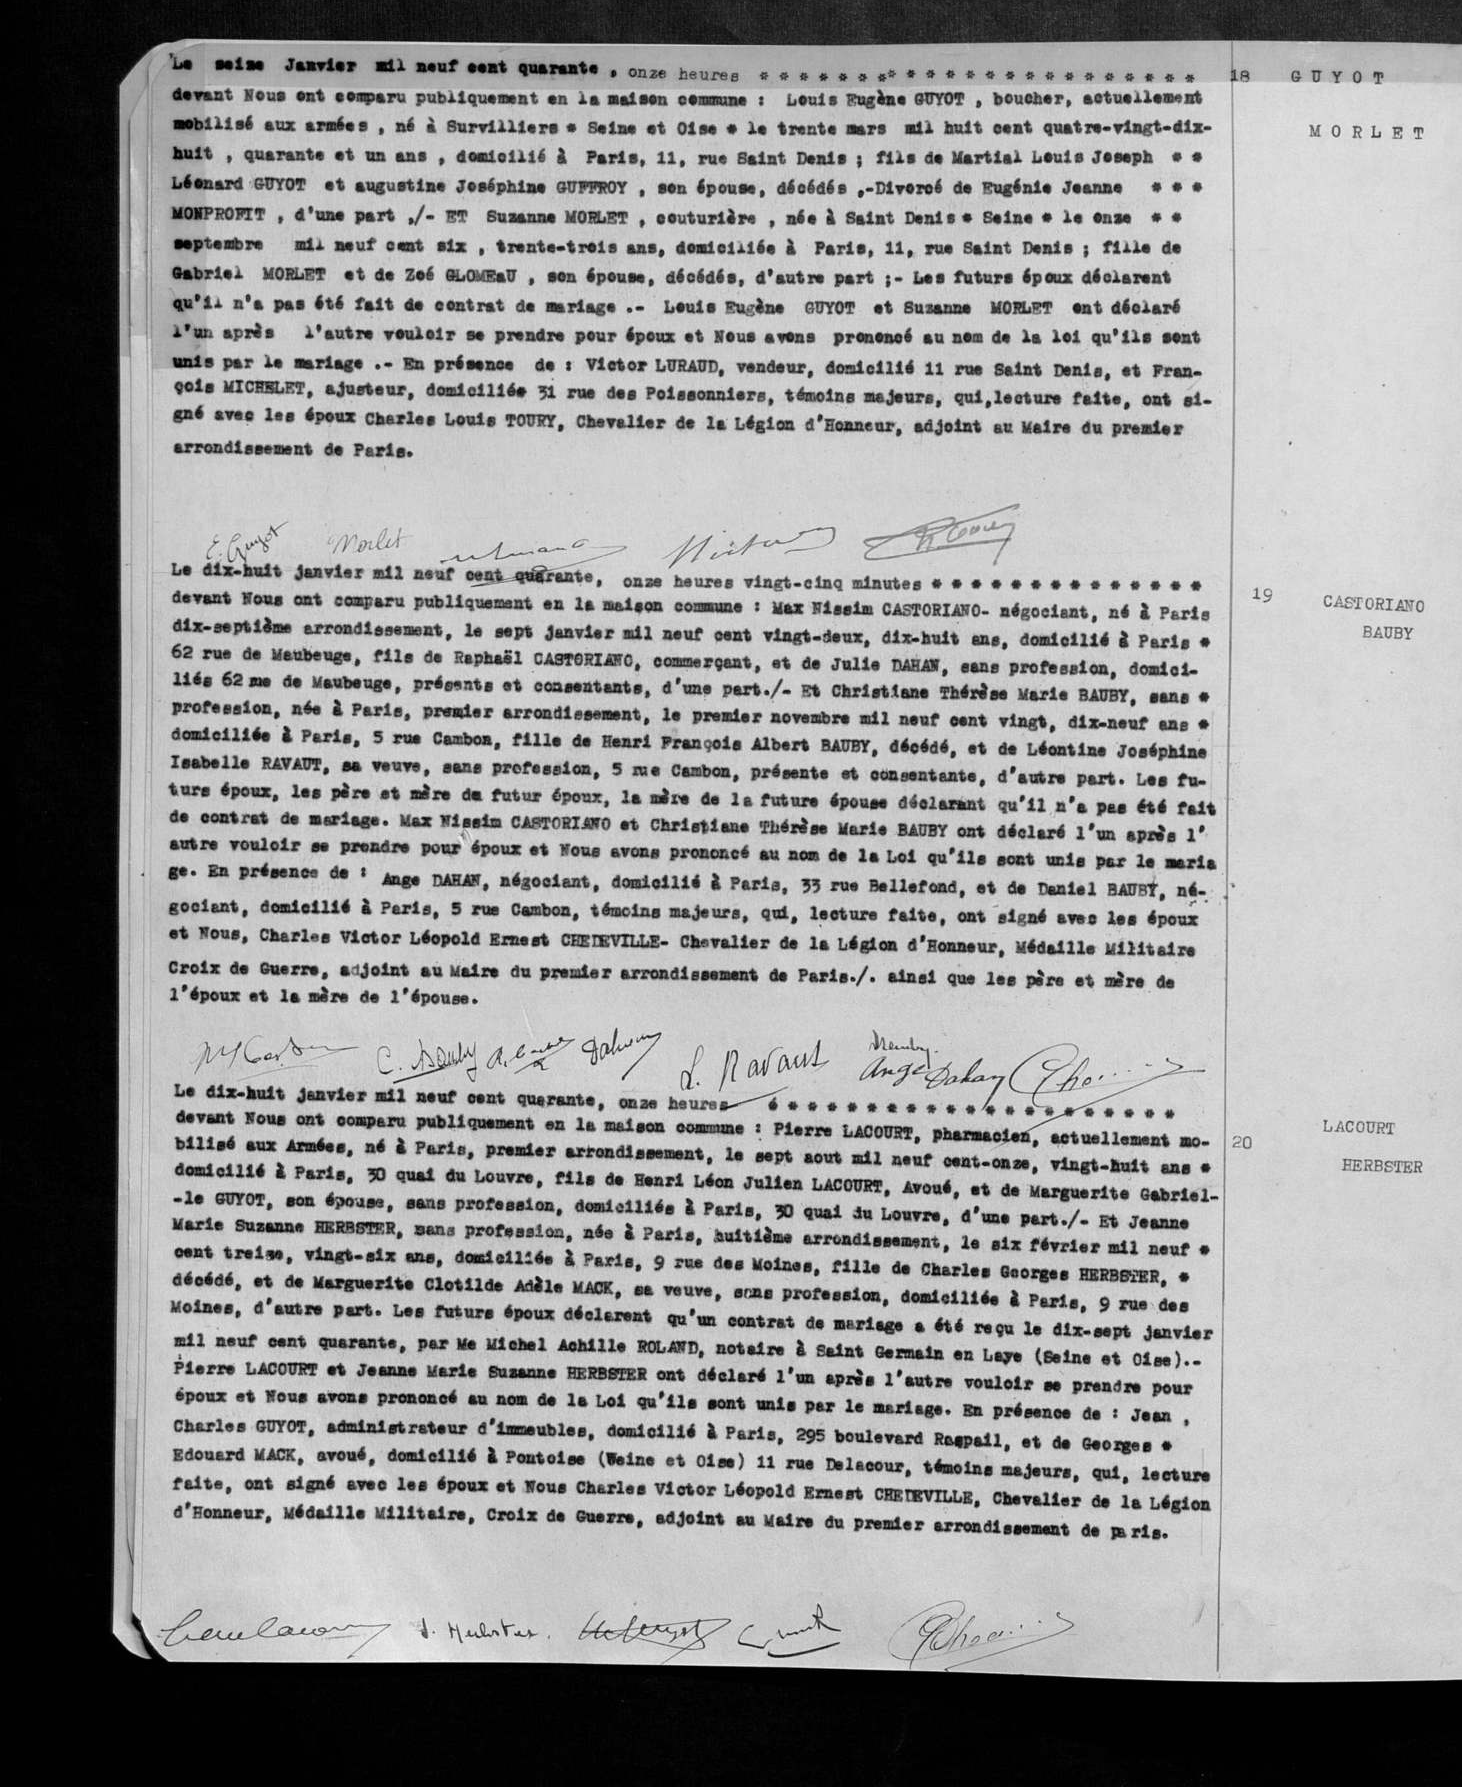Le seize janvier mil neuf cent quarante, onze heures ****
devant Nous ont comparu publiquement en la maison commune: Louis Eugène GUYOT, boucher, actuellement
mobilisé aux armées, né à Suvrilliers * Seine et Oise * le trente mars mil huit cent quatre-vingt-dix-
huit, quarante et un ans, domicilié à Paris, 11, rue Saint Denis; fils de Martial Louis Joseph **
Léonard Guyot et augustine Joséphine GUFFROY, son épouse, décédés ,-Divorcé de Eugénie Jeanne ***
MONPROFIT, d'une part ,/-ET Suzanne MORLET, couturière, née à Saint Denis * Seine * le onze **
septembre mil neuf cent six, trente-trois ans, domiciliée à Paris, 11, rue Saint Denis; fille de
Gabriel MORLET et de Zoé GLOMaU, son épouse, décédés, d'autre part ;-Les futurs époux déclarent
qu'il n'a pas été fait de contrat de mariage .-Louis Eugène GUYOT et Suzanne MORLET ont déclaré
l'un après l'autre vouloir se prendre pour époux et Nous avons prononcé au nom de la loi qu'ils sont
unis par le mariage .-En présence de: Victor LURAUD, vendeur, domicilié 11 rue Saint Denis, et Fran-
cois MICHELET, ajusteur, domiciliée 31 rue des Poissonniers, témoins majeurs, qui, lecture faite, ont si-
gné avec les époux Charles Louis TOURY, Chevalier de la Légion d'Honneur, adjoint au Maire du premier
arrondissement de Paris.
Le dix-huit janvier mil neuf cent quarante, onze heures vingt-cinq minutes ****
devant Nous ont comparu publiquement en la maison commune: Max Nissim CASTORIANO-négociant, né à Paris
dix-septième arrondissement, le sept janvier mil neuf cent vingt-deux, dix-huit ans, domicilié à Paris *
62 rue de Maubeuge, fils de Raphaël CASTORIANO, commerçant, et de Julie DAHAN, sans profession, domici-
liés 62 rue de Maubeuge, présent et constants, d'une part ./-Et Christiane Thérèse Marie BAUBY, sans *
profession, née à Paris, premier arrondissement, le premier novembre mil neuf cent vingt, dix-neuf ans *
domiciliée à Paris, 5 rue Cambon, fille de Henri François Albert BAUBY, décédé, et de Léontine Joséphine
Isabelle RAVAUT, sa veuve, sans profession, 5 rue Cambon, présente et consentante, d'autre part. Les fu-
turs époux, les pères et mères du futur époux, la mère de la future épouse déclarent qu'il n'a pas été fait
de contrat de mariage. Max Nissim CASTORIANO et Christiane Thérèse Marie BAUBY ont déclaré l'un après l'
autre vouloir se prendre pour époux et Nous avons prononcé au nom de la Loi qu'ils sont unis par le maria
ge. En présence de: Ange DAHAN, négociant, domicilié à Paris, 33 rue Bellefond, et de Daniel BAUBY, né-
gociant, domicilié à Paris, 5 rue Cambon, témoins majeurs, qui, lecture faite, ont signé avec les époux
et Nous, Charles Victor Léopold Ernest, CHELEVILLE-Chevalier de la Légion d'Honneur, Médaille Militaire
Croix de Guerre, adjoint au Maire du premier arrondissement de Paris ./. ainsi que les père et mère de
l'époux et la mère de l'épouse.
Le dix-huit janvier mil neuf cent quarante, onze heures ****
devant Nous ont comparu publiquement en la maison commune: Pierre LACOURT, pharmacien, actuellement mo-
bilisé aux Armées, né à Paris, premier arrondissement, le sept aout mil neuf cent-onze, vingt-huit ans *
domicilié à paris, 30 quai du Louvre, fils de Henri Léon Julien LACOURT, Avoué, et de Marguerite Gabriel-
-le GUYOT, son épouse, sans profession, domiciliée à Paris, 30 quai du Louvre, d'une part ./-Et Jeanne
Marie Suzanne HERBSTER, sans profession, née à Pairs, huitième arrondissement, le six février mil neuf *
cent treize, vingt-six ans, domiciliées à Paris, 9 rue des Moines, fille de Charles Georges HERNSTER, *
décédé, et de Marguerite Clotilde Adèle MACK, sa veuve, sans profession, domiciliée à Paris, 9 rue des
Moines, d'autre part. Les futurs époux déclarent qu'un contrat de mariage a été reçu le dix-sept janvier
mil neuf cent quarante, par Me Michel Achille ROLAND, notaire à Saint Germain en Laye (Seine et Oise) .-
Pierre LACOURT et Jeanne Marie Suzanne HERBSTER ont déclaré l'un après l'autre vouloir se prendre pour
époux et Nous avons prononcé au nom de la Loi qu'ils sont unis par le mariage En présence de: Jean,
Charles GUYOT, administrateur d'immeubles, domicilié à Paris, 295 boulevard Raspail, et de Georges *
Edouard MACH, avoué, domicilié à Pontoise (Veine et Oise) 11 rue Delacour, témoins majeurs, qui, lecture
faite, ont signé avec les époux et Nous Charles Victor Léopold Ernest CHELEVILLE, Chevalier de la Légion
d'Honneur, Médaille Militaire, Croix de Guerre, adjoint au Maire du premier arrondissement de paris.
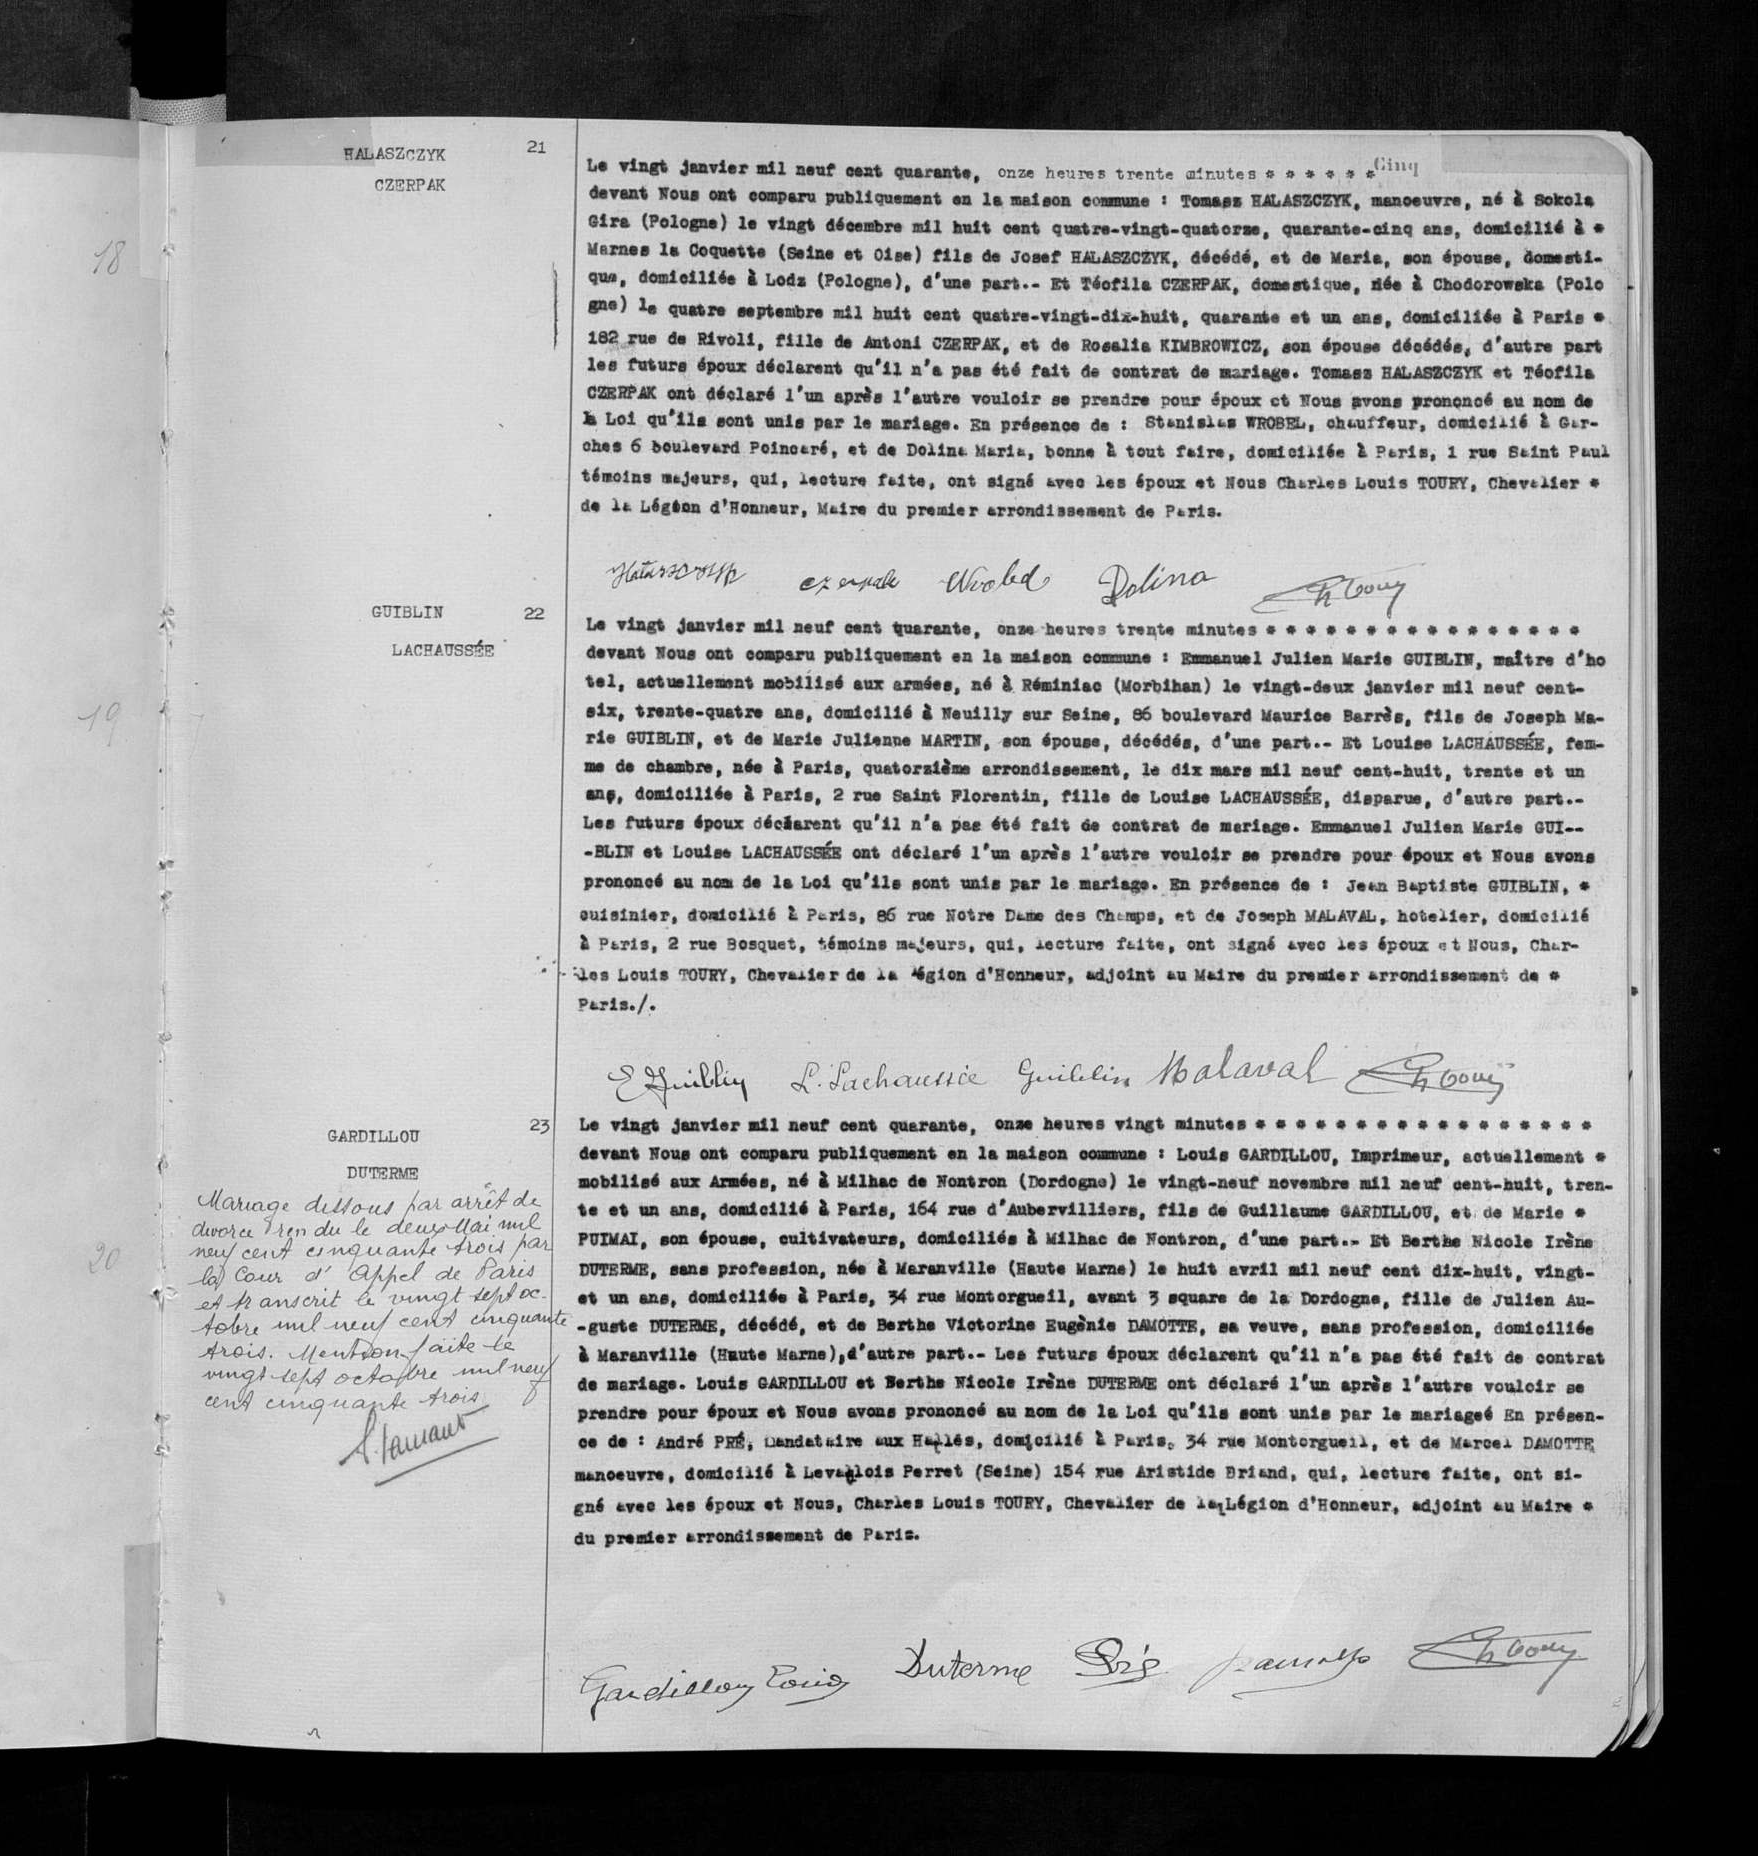Le vingt janvier mil neuf cent quarante, onze heures trente minutes ****
devant Nous ont comparu publiquement en la maison commune: Tomasz HALASZCZYK, manoeuvre, né à Sokola
Gira (Pologne) le vingt décembre mil huit cent quatre-vingt-quatorze, quarante-cinq ans, domicilié à *
Marnes La Coquette (Seine et Oise) fils de Joseph HALASZCZYK, décédé et de Maria, son épouse domesti-
que, domiciliée à Lodz (Pologne), d'une part.-Et Téofila CZERPAK, domestique, née à Chodorwaka (Polo
gne) le quatre septembre mil huit cent quatre-vingt-dix-huit, quarante et un ans, domiciliée à Paris *
182 rue de Rivoli, fille de Antoni OZERPAK, et de Rosalie KIMBROWICK, son épouse décédés, d'autre part
les futurs époux déclarent qu'il n'a pas été fait de contrat de mariage. Tomasz HALASZCZYK et Téofila
CZERPAK ont déclaré l'un après l'autre vouloir se prendre pour époux et Nous avons prononcé au nom de
la Loi qu'ils sont unis par le mariage. En présence de: Sanislas WROBEL, chauffeur, domicilié à Gar-
ces 6 boulevard Poincaré, et de Dolina Maria, bonne à tout faire, domiciliée à Paris, 1 rue Saint Paul
témoins majeurs, qui, lecture faite, ont signé avec les époux et Nous Charles Louis TOURY, chevalier *
de la Légion d'Honneur, Maire du premier arrondissement de Paris.
Le vingt janvier mil neuf cent quarante, onze heures trente minutes ****
devant Nous ont comparu publiquement en la maison commune: Emmanuel Julien Marie GUIBLIN, maître d'ho
tel, actuellement mobilisé aux armées, né à Réminiac (Morbihan) le vingt-deux janvier mil neuf cent-
six, trente-quatre ans, domicilié à Neuilly sur Seine, 96 boulevard Maurice Barrès, fils de Joseph Ma-
rie GUIBLIN, et de Marie Julienne MARTIN, son épouse, décédées, d'une part.-Et Louise LACHAUSSEE, fem-
me de chambre, née à Paris, quatorzième arrondissement, le dix mars mil neuf cent-huit, trente et un
ans, domiciliée à Paris, 2 rue Saint Florentin, fille de Louise LACHAUSSEE, disparue, d'autre part.-
Les futurs époux déclarent qu'il n'a pas été fait de contrat de mariage. Emmanuel Julien Marie GUI-
BLIN et Louise LACHAUSSEE ont déclaré l'un après l'autre vouloir se prendre pour époux et Nous avons
prononcé au nom de la Loi u'ils sont unis par le mariage. En présence de: Jean Baptise GUIBLIN, *
cuisinier, domicilié à Paris, 86 rue Notre Dame des Champs, et de Joseph MALAVAL, hôtelier, domicilié
à Paris, 2 rue Bosquet, témoins majeurs, qui, lecture faite ont signé avec les époux e t Nous, Char-
les Louis TOURY, Chevalier de la légion d'Honneur, adjoint au maire du premier arrondissement de *
Paris ./.
Le vingt janvier mil neuf cent quarante, onze heure vingt minutes ****
devant Nous ont comparu publiquement en la maison commune: Louis GARDILLOU, Imprimeur, actuellement *
mobilisé aux Armées, né à Milhac de Nontron (Dordogne) le vingt-neuf novembre mil neuf cent-huit, tren-
te et un ans, domicilié à Paris, 164 rue d'Aubervilliers, fils de Guillaume GARDILLOU, et de Marie *
PUIMAI, son épouse, cultivateurs, domiciliés à Milhac de Nontron, d'une part .-ET Berthe Nicole Irène
DUTERME, sans profession, née à Maranville (Haute Marne) le huit avril mil neuf cent dix-huit, vingt-
et un ans, domicilié à Paris, 34 rue Montorgeuil, avant 3 square de la Dordogne, fille de Julien Au-
-guste DUTERME, décédé, et de Berthe Victorine Eugènie DAMOTTE, sa veuve, sans profession, domiciliée
à Maranville (Haute Marne), d'autre part .-Les futurs époux déclarent qu'il n'a pas été fait de contrat
de mariage Louis GARDILLOU et Berte Nicole Irène DUTERME ont déclaré l'un après l'autre vouloir se
prendre pour époux et Nous avons prononcé au nom de la Loi qu'ils sont unis par le mariageé En présen-
ce de: André PRE, Dandataire aux Halles, domicilié à Paris, 34 rue Montorgueuil, et de Marcel DAMOTTE
manoeuvre, domicilié à Levallois Perret (Seine) 154 rue Aristide Briand, qui, lecture faite, ont si-
gné avec les époux et Nous, Charles Louis TOURY, Chevalier de la Légion d'Honneur, adjoint au Maire *
du premier arrondissement de Paris.